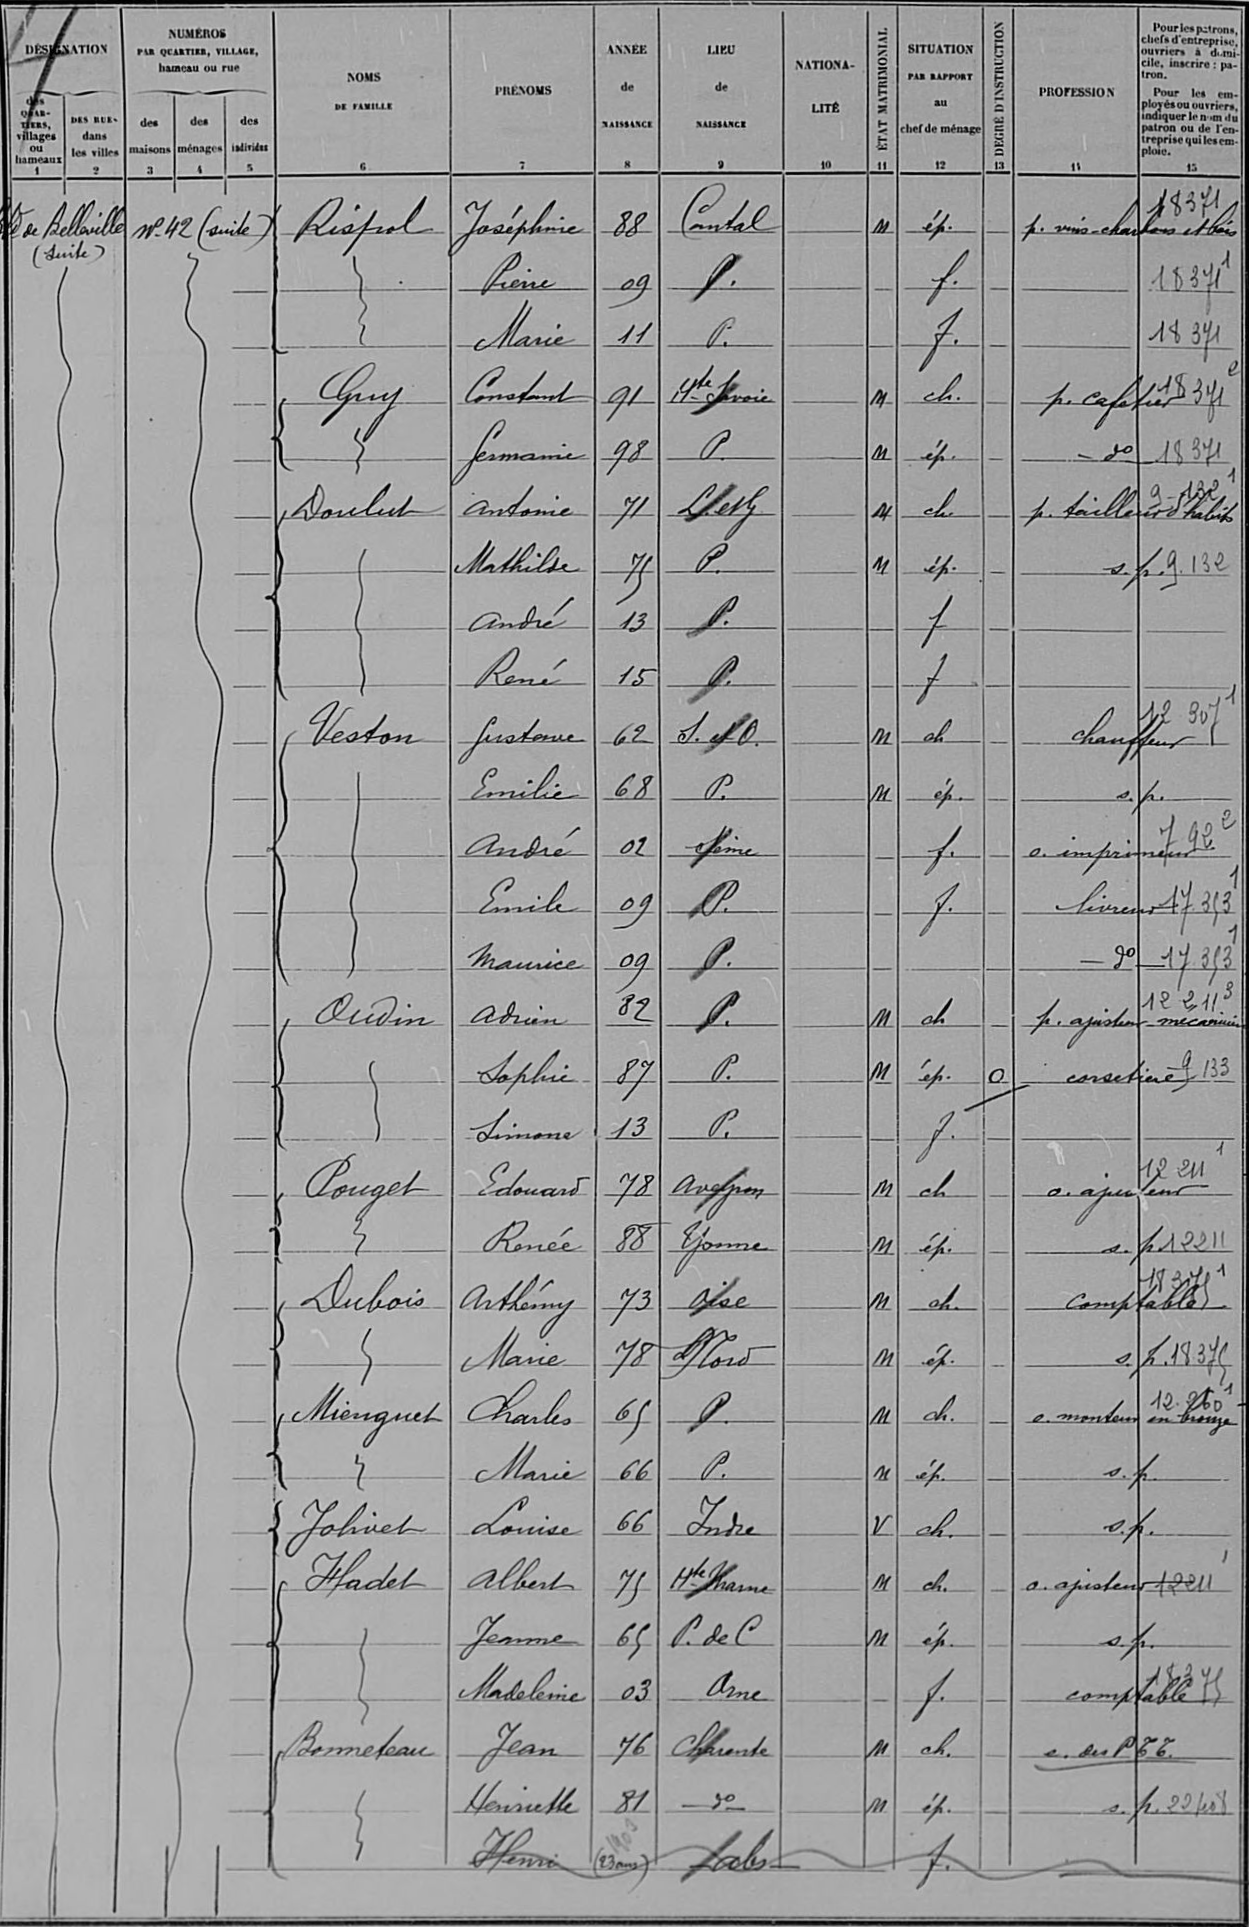Rispol/Joséphine/88/Cantal/¤/M/ép /¤/p vins-charbons et bois/¤
¤/Pierre/09/P /¤/¤/f /¤/¤/183711
¤/Marie/11/P /¤/¤/f /¤/¤/183712
Guy/Constant/91/Hte Savoie/¤/M/ch /¤/p cafetier/183712
¤/Germaine/98/P /¤/M/ép /¤/d°/183711
Doulut/Antoine/71/L et G/¤/M/ch /¤/p tailleur d'habits/¤
¤/mathilde/75/P /¤/M/ép /¤/s p /9132
¤/André/13/P /¤/¤/f/¤/¤/¤/¤
¤/René/15/P /¤/¤/f/¤/¤/1/¤
Veston/Gustave/62/S et O /¤/M/ch/¤/chauffeur/¤
¤/Emilie/68/P /¤/M/ép /¤/s p /¤/¤
¤/André/02/Seine/¤/¤/f /¤/o imprimeur/¤
¤/Emile/09/P /¤/¤/f /¤/livreur/17 3531
¤/Maurice/09/P /¤/¤/¤/¤/d°/173531
Oudin/Adrien/82/P /¤/M/ch/¤/p ajusteur mecanicien/¤
¤/Sophie/87/P /¤/M/ép /O/corsetiere/9133
¤/Simone/13/P /¤/¤/f /¤/¤/1/¤
Pouget/Edouard/78/Aveyron/¤/M/ch/¤/o ajusteur/¤
¤/Renée/88/Yonne/¤/M/ép /¤/s p /122111
Dubois/Arthémy/73/Oise/¤/M/ch /¤/comptable/¤
¤/Marie/78/Nord/¤/M/ép /¤/s p /18375
Mieuguet/Charles/65/P /¤/M/ch /¤/o monteur en bronze/¤
¤/Marie/66/P /¤/M/ép /¤/s p /¤/¤
Jolivet/Louise/66/Indre/¤/V/ch /¤/s p /¤/¤
Hadet/Albert/75/Hte Marne/¤/M/ch /¤/o ajusteur/12211
¤/Jeanne/65/P de C/¤/M/ép /¤/s p /¤/¤
¤/Madeleine/03/Orne/¤/¤/f /¤/comptable/¤
Bonneteau/Jean/76/Charente/¤/M/ch /¤/e du PTT/¤/¤
¤/Henriette/81/d°/¤/M/ép /¤/s p /22408
¤/Henri/(23 ans)/abs/¤/¤/f /¤/¤/¤/¤
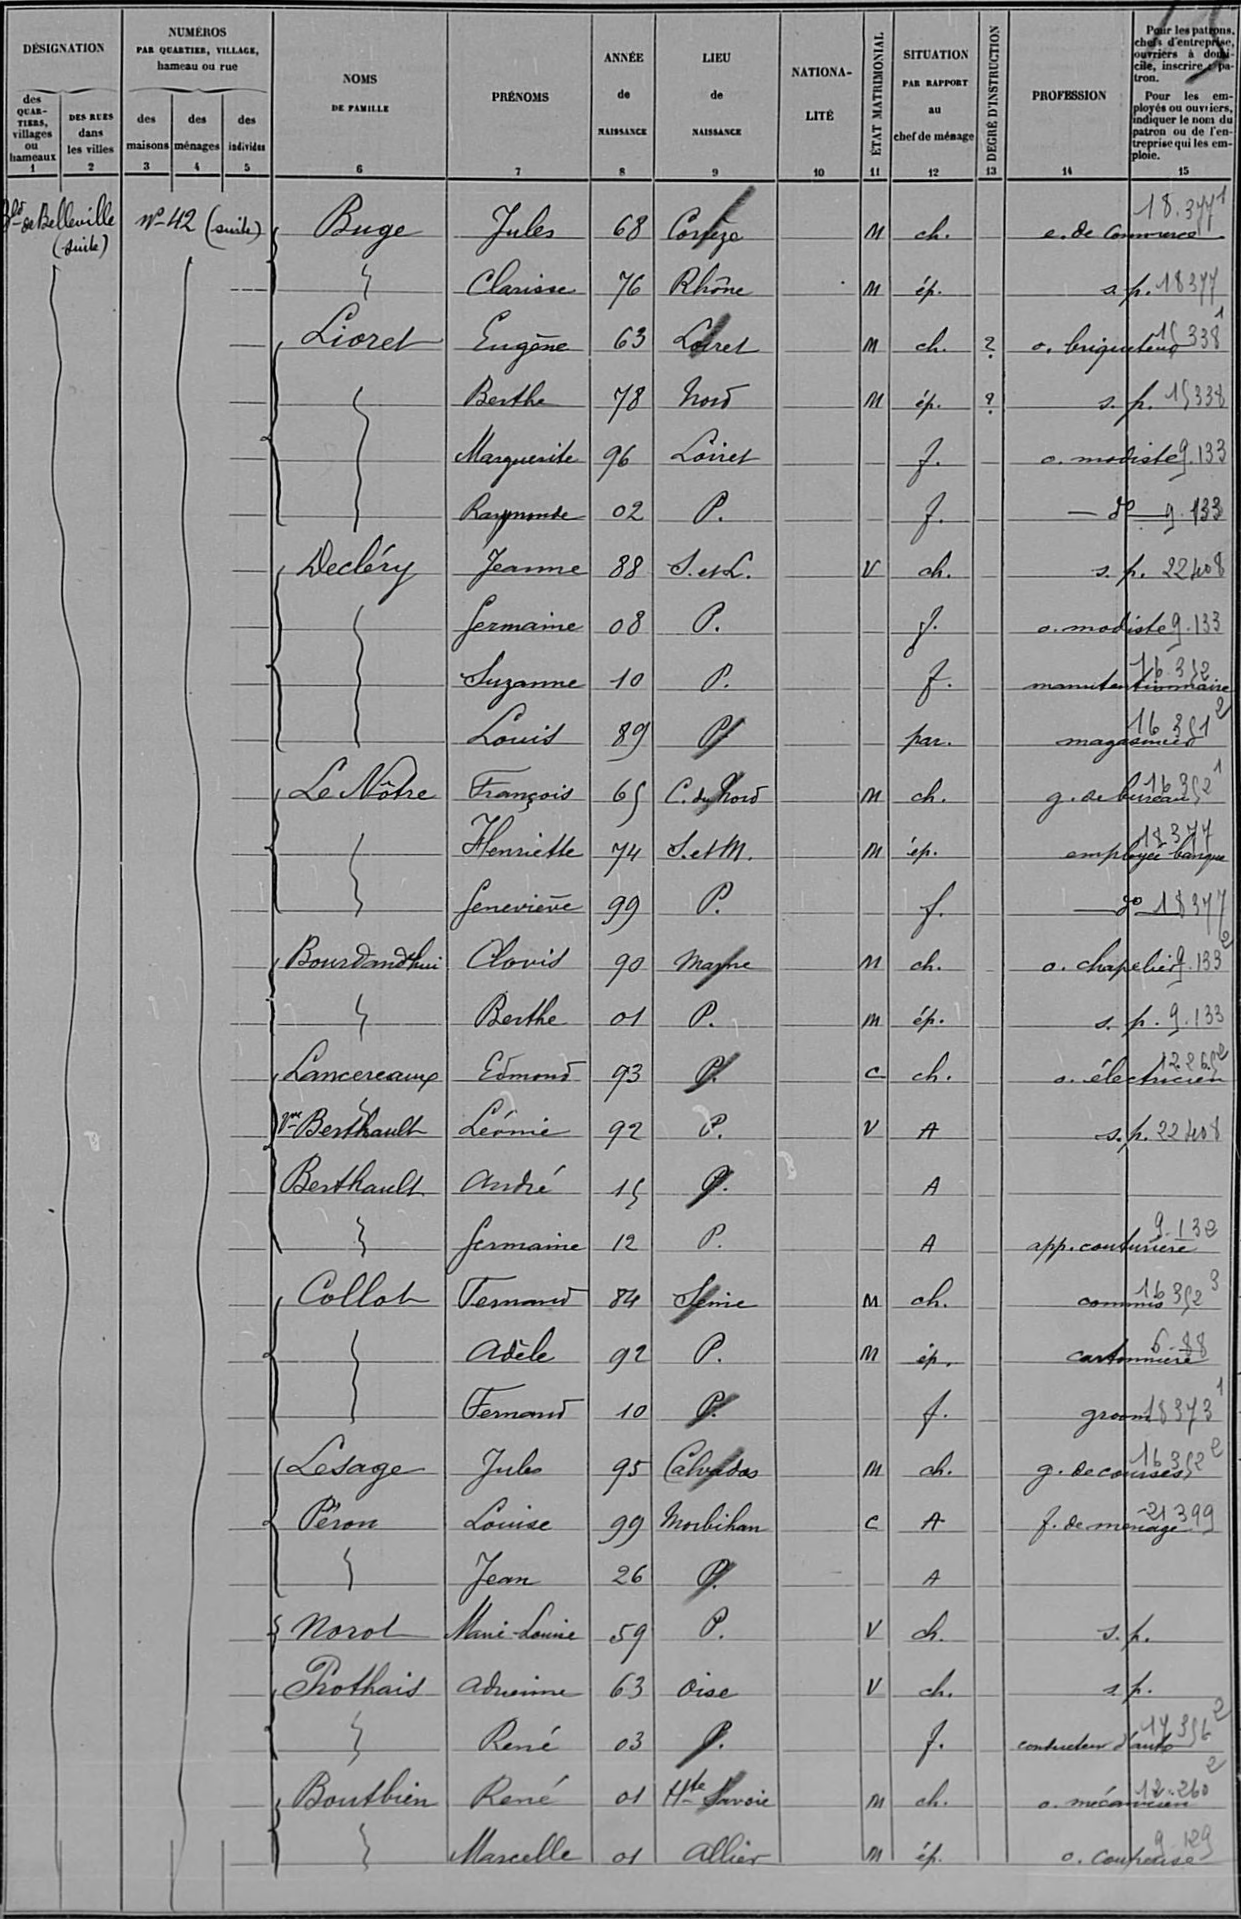Buge/Jules/68/Corrèze/¤/M/ch /¤/e de commerce/183771
¤/Clarisse/76/Rhône/¤/M/ép /¤/s pr /18377
Lioret/Eugène/63/Loiret/¤/M/ch /¤/o briqueteur/¤
¤/Berthe/78/Nord/¤/M/ép /¤/s pr /15338
¤/Marguerite/96/Loiret/¤/¤/f /¤/o modiste/9 133
¤/Raymonde/02/P /¤/¤/f /¤/d°/9 133
Decléry/Jeanne/88/S et L /¤/V/ch /s p /22408
¤/Germaine/08/P /¤/¤/f /¤/o modiste/9133
¤/Suzanne/10/P /¤/¤/f /¤/manutentionnaire/¤
¤/Louis/89/P /¤/¤/par /¤/magasinier/¤
Le Nôtre/François/65/C du Nord/¤/M/ch /¤/g de bureau/¤
¤/Henriette/74/S et M /¤/M/ép /¤/employée banque/¤
¤/Geneviève/99/P /¤/¤/f /¤/d°/183772
Bourvand'hui/Clovis/90/Marne/M/ch /¤/o chapelier/9 1332
¤/Berthe/01/P /¤/M/ép /¤/s pr /9 133
Lancereaux/Edmond/93/P /¤/c/ch /¤/o électricien/¤
Vve Berthault/Léonie/92/P /¤/V/A/¤/s p /22408
Berthault/André/15/P /¤/¤/A/¤/¤/¤/¤
¤/Germaine/12/P /¤/¤/A/¤/app couturière/9 132
Collot/Fernand/84/Seine/¤/M/ch /¤/commis/163523
¤/Adèle/92/P /¤/M/ép /¤/cartonnière/6 88
¤/Fernand/10/P /¤/¤/f /¤/groom/183731
Lesage/Jules/95/Calvados/¤/M/ch /¤/g de courses/¤
Péron/Louise/99/Morbihan/¤/C/A/¤/f de ménage/21399
¤/Jean/26/P /¤/¤/A/¤/¤/¤/¤
Norot/Marie-Louise/59/P /¤/V/ch /¤/s p /¤/¤
Prothais/Adrienne/63/Oise/¤/V/ch /¤/s p /2/¤
¤/René/03/P /¤/¤/f /¤/conducteur d'auto/¤
Boutbien/René/01/Hte Savoie/¤/M/ch /¤/o mécanicien/¤
¤/Marcelle/01/Allier/¤/M/ép /¤/o coupeuse/¤
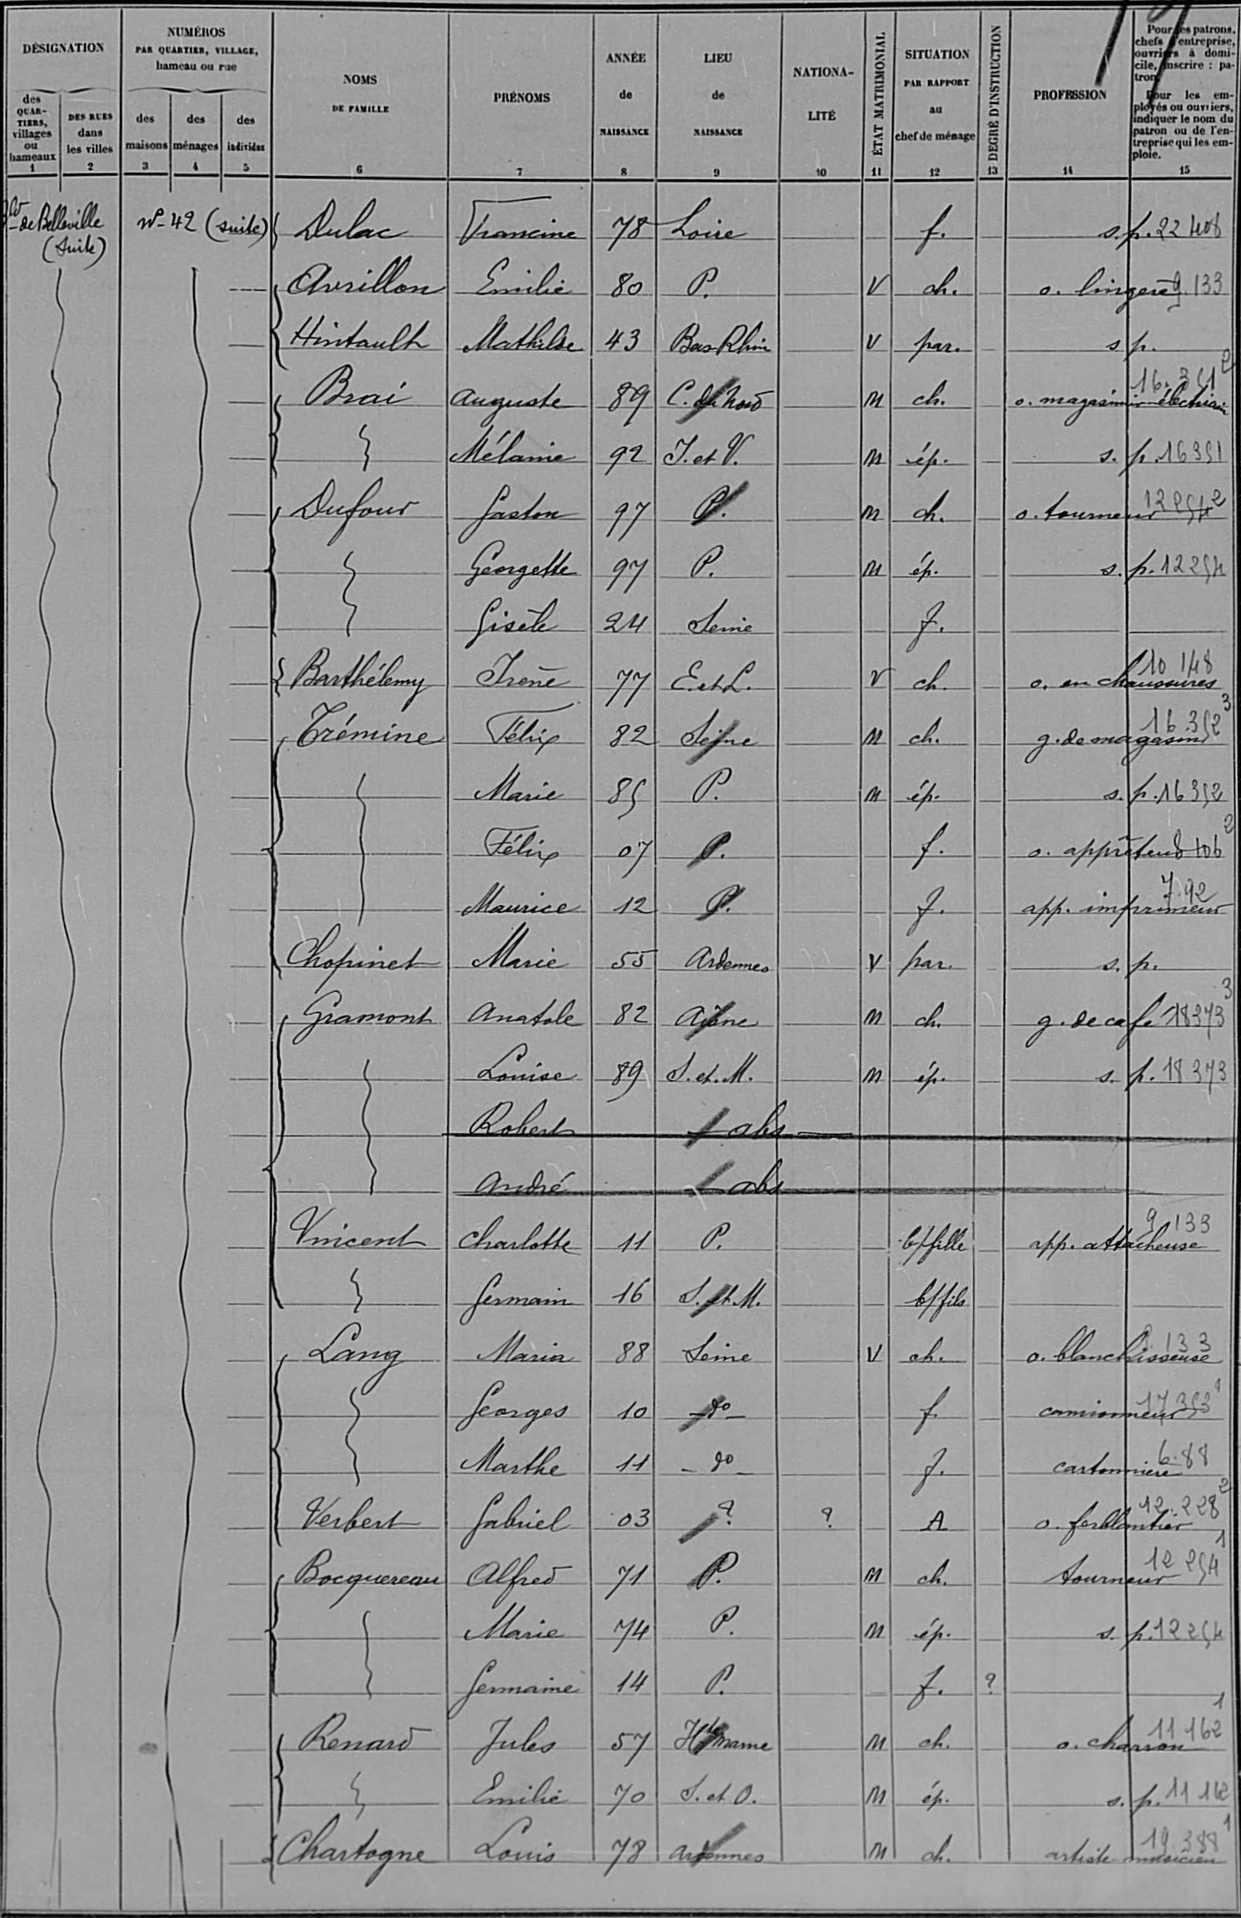Dulac/Francine/78/Loire/¤/¤/f /¤/s p /22408
Avrillon/Emilie/80/P /¤/V/ch /¤/o lingère/9133
Hintault/Mathilde/43/Bas-Rhin/¤/V/par /¤/s p /¤/¤
Brai/Auguste/89/C du Nord/M/ch /¤/o magasinier-électricien/¤
¤/mélanie/92/I et V /¤/M/ép /¤/s p /16351
Dufour/Gaston/97/P /¤/m/ch /¤/o tourneur/122512
¤/Georgette/97/P /¤/M/ép /¤/s p /12254
¤/Gisèle/24/Seine/¤/¤/f /¤/¤/¤/¤
Barthélemy/Irène/77/E et L /¤/V/ch /¤/o en chaussures/¤
Crémine/Félix/82/Seine/¤/M/ch /¤/g de magasin/¤
¤/Marie/85/P /¤/M/ép /¤/s pr /163522
Félix/07/P /¤/¤/f /¤/o apprêteur/81062
¤/Maurice/12/P /¤/¤/f /¤/o imprimeur/¤
Chopinet/Marie/55/Ardennes/¤/V/par /¤/s p /3
Gramont/Anatole/82/Aisne/¤/M/ch /¤/g de café/183733
¤/Louise/89/S et M /¤/M/ép /¤/s p /18373
¤/Robert/¤/abs/¤/¤/¤/¤/¤/¤/¤
¤/André/¤/abs/¤/¤/¤/¤/¤/¤/¤
Vincent/Charlotte/11/P /¤/¤/b-fille/¤/app attacheuse/¤
¤/Germain/16/S et M /¤/¤/b-fils/¤/¤/¤/¤
Lang/Maria/88/Seine/¤/V/ch /¤/o blanchisseuse/¤
¤/Georges/10/d°/¤/¤/f /¤/camionneur/173531
¤/Marthe/11/d°/¤/¤/f /¤/cartonnière/6 882
Verbert/Gabriel/03/¤/¤/¤/A/¤/o ferblantier/¤
Bocquereau/Alfred/71/P /¤/M/tourneur/¤
¤/Marie/74/P /¤/M/ép /¤/s p /12254
¤/Germaine/14/P /¤/¤/f /¤/¤/1
Renard/Jules/57/Hte Marne/¤/M/ch /¤/o charron/¤
¤/Emilie/70/S et O /¤/M/ép /¤/s p /11112
Chartogne/Louis/78/Ardennes/¤/M/ch /¤/artiste musicien/¤